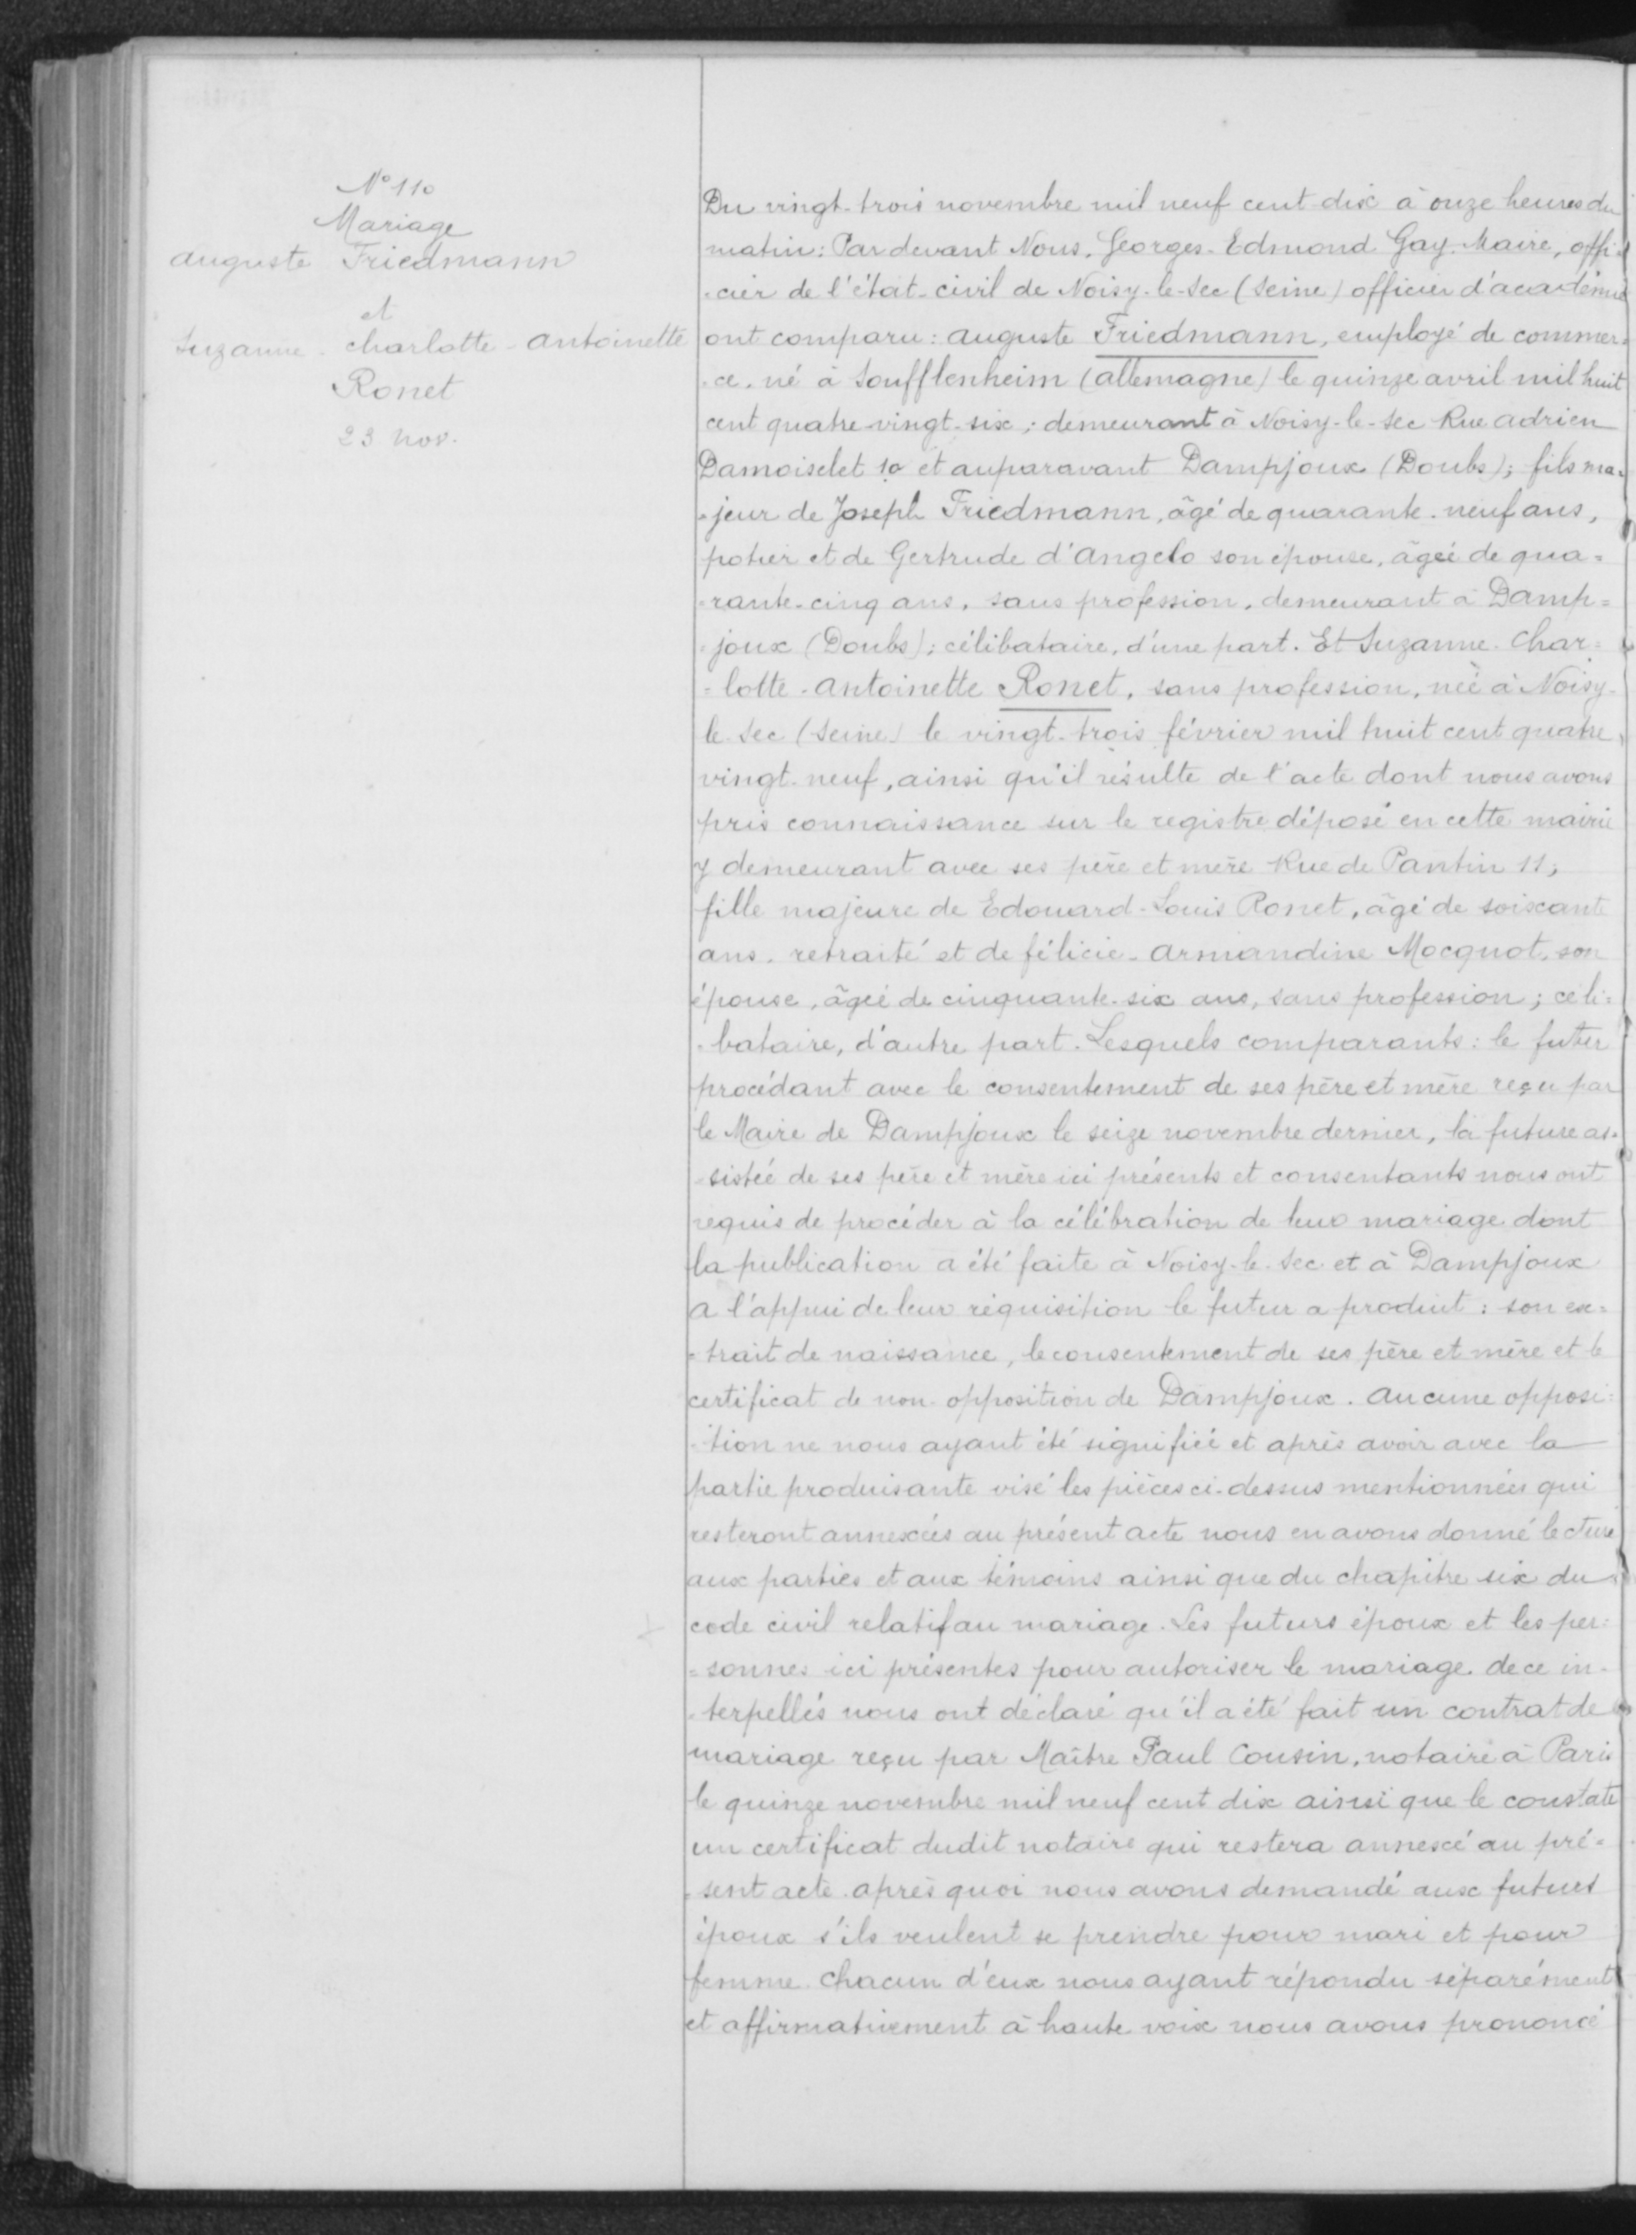N° 110
Mariage
Auguste Friedmann
et
Suzanne Charlotte-Antoinette
Ronet
23 nov
Du vingt-trois novembre mil neuf cent dix à onze heures du
matin: Par devant Nous. Georges Edmond Gay Maire offi-
-cier de l'état-civil de Noisy-le-sec (Seine) officier d'académie
ont comparu: Augste Friedmann, employé de commer
-ce, né à Soufflenheim (Allemagne) le quinze avril mil huit
cent quatre vingt six; femeurant à Noisy-le-sec Rue Adrien
Damoiselet 10 et auparavant Dampjoux (Doubs); fils ma-
-jeur de Joseph Friedmann, âgé de quarante-neuf ans,
potier et de Gertrude d'Angelo son épouse, âgée de qua-
-rante-cinq ans, sans profession, demeurant à Damp-
joux (Doubs); célibataire, d'une part. Et Suzanne Char
lotte-Antoinette Ronet, sans profession, née à Noisy-
le-Sec (Seine) le vingt-trois février mil huit-cent quatre
vingt-neuf, ainsi qu'il résulte de l'acte dont nous avons
pris connaissance sur le registre déposé en cette mairie
y demeurant avec ses père et mère Rue de Pantin 11;
fille majeure de Edouard-Louis Ronet, âgé de soixante
ans-Retraité et de félicie-Armandine Mocquot, son
épouse, âgée de cinquante-six ans, sans profession; céli
-bataire, d'autre part. Lesquels comparantes: le futur
procédant avec le consentement de ses père et mère reçu par
le Maire de Dampjoux le seize novembre dernier, la futur as-
sistée de ses père et mère ici présents et consentants nous ont
requis de procéder à la célébration de leur mariage dont
la publication a été faite à Noicy-le-Sec et à Dampjoux
A l'appui de leur requisition le futur a produit: son ex-
-trait de naissance, le consentement de ses père et mère et le
certificat de non-opposition de Dampjoux. Aucune opposi-
-tion ne nous ayant été signifié et après avoir avec la
partie produisanté visé les pièces ci-dessus mentionnées qui
resteront annexées au présent acte nous en avons donné lecture
aux parties et aux témoins ainsi que du chapitre six du
code civil relatif au mariage. Les futurs époux et les per-
sonnes ici présentes pour autoriser le mariage. de ce in-
-terpellés nous ont déclaré qu'il a été fait un contrat de
mariage reçu par Maitre Paul Cousin, notaire à Paris
le quinze novembre mil neuf cent dix ainsi que le constate
un certificat dudit notaire qui restera annexé au pré-
sent acte après quoi nous avons demandé aux futurs
époux s'ils veulent se prendre pour mari et pour
femme. Chacun d'eux nous ayant répondu séparément
et affirmativement à haute voix nous avons prononcé
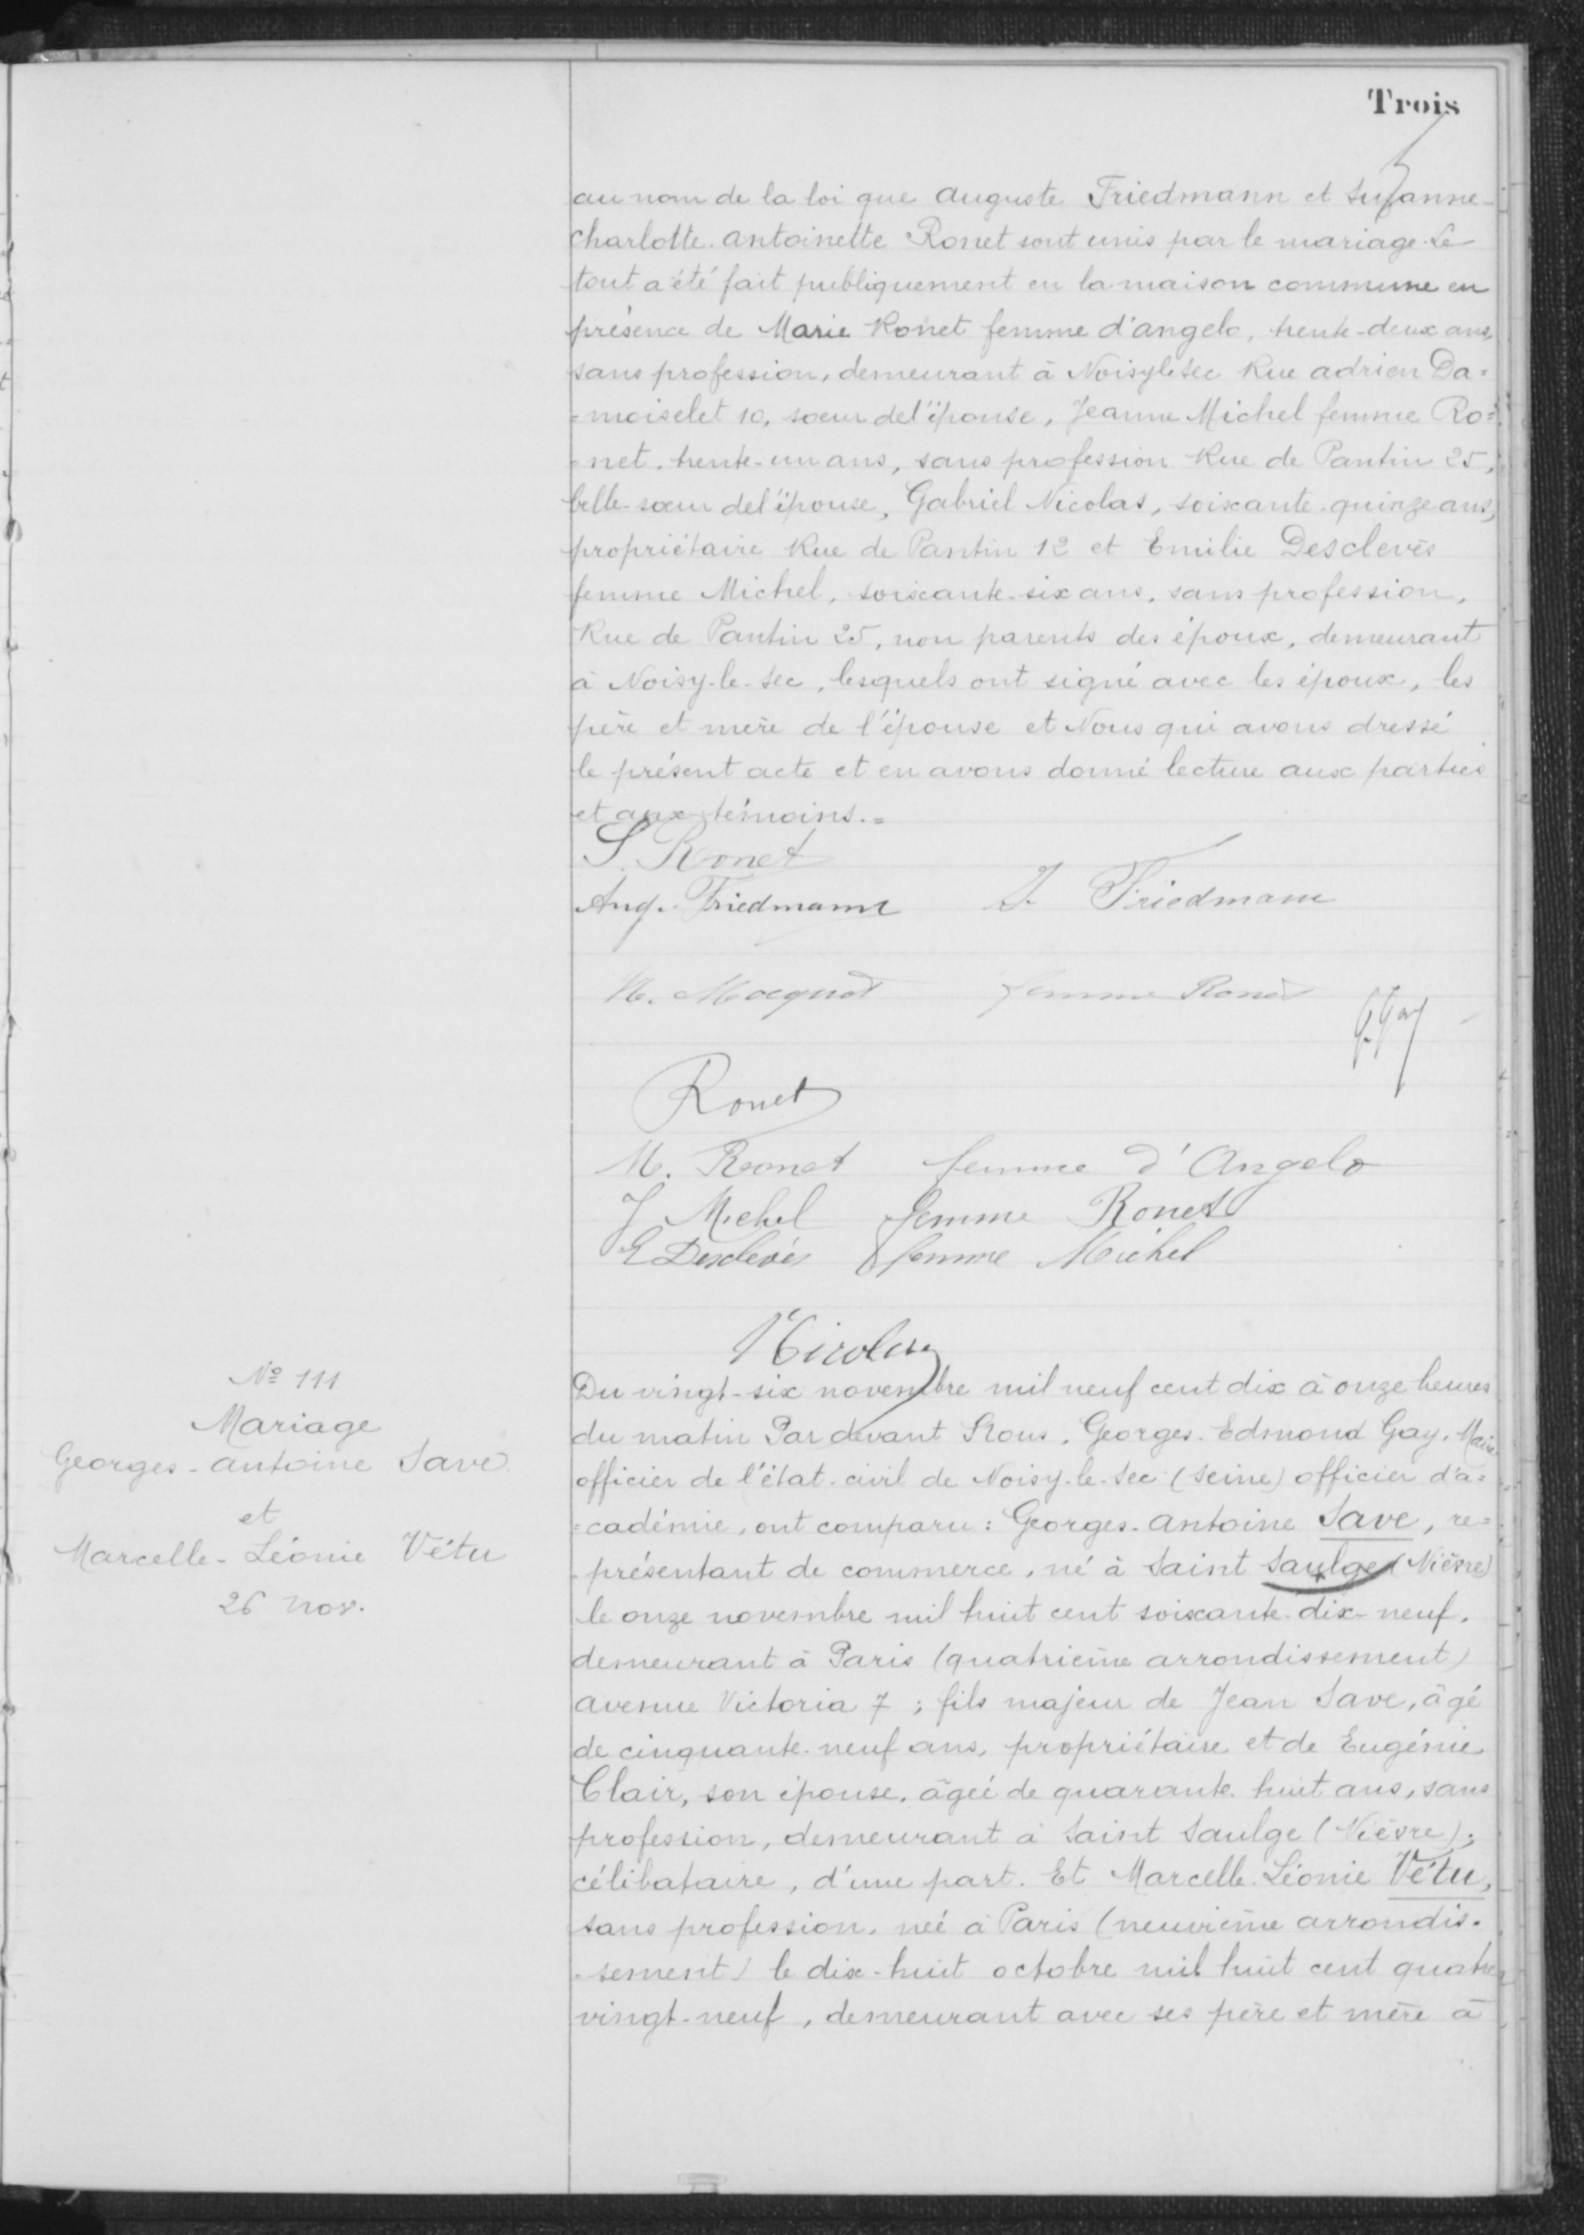au nom de la loi que Auguste Friedmann et Suzanne
Charlotte Antoinette Ronet sont unis par le mariage. Le
tout a été fait publiquement en la maison commune en
présence de Marie Ronet femme d'angelo, trente-deux ans
sans profession, demeurant à Noisy-le-sec Rue adrien Da-
-moiselet 10, soeur del'épouse, Jeanne Michel femme Ro
-net, trente-un ans, sans profession Rue de Pantin 25
belle soeur del'épouse, Gabriel Nicolas, soixante-quinze ans,
propriétaire Rue de Pantin 12 et Emilie Desclevés
femme Michel, soixante-six ans, sans profession
Rue de Pantin 25, non parents des époux, demeurant
à Noisy-le-sec, lesquels ont signé avec les époux, les
père et mère de l'épouse et Nous qui avons dressé
Le présent acte et en avons donné lecture aux parties
et aux témoins.
N° 111
Mariage
Georges-Antoine Save
et
Marcelle-Léonie Vétu
26 nov
Du vingt-six novembre mil neuf cent dix à onze heures
du matin Par devant Nous. Georges Admond Gay. Maire
officier de l'état-civil de Noisy-le-sec (seine) officier d'a
cadémie, ont comparu: Georges Antoine Save, re
-présentant de commerce, né à Saint Saulge (Nièves)
le onze novembre mil huit cent soixante-dix-neuf
demeurant à Paris (quatrième arrondissement)
avenue Victoria 7; fils majeur de Jean Save, âgé
de cinquante-neuf ans, propriétaire et de Eugénie
Clair, son épouse, âgée de quarante-huit ans, sans
profession, demeurant à Saint Saulge (Nièvre)
célibataire, d'une part. Et Marcelle Léonie Vétu
sans profession, né à Paris (neuvième arrondis-
-sement) le dix-huit octobre mil huit cent quatre
vingt-neuf, demeurant avec ses père et mère à